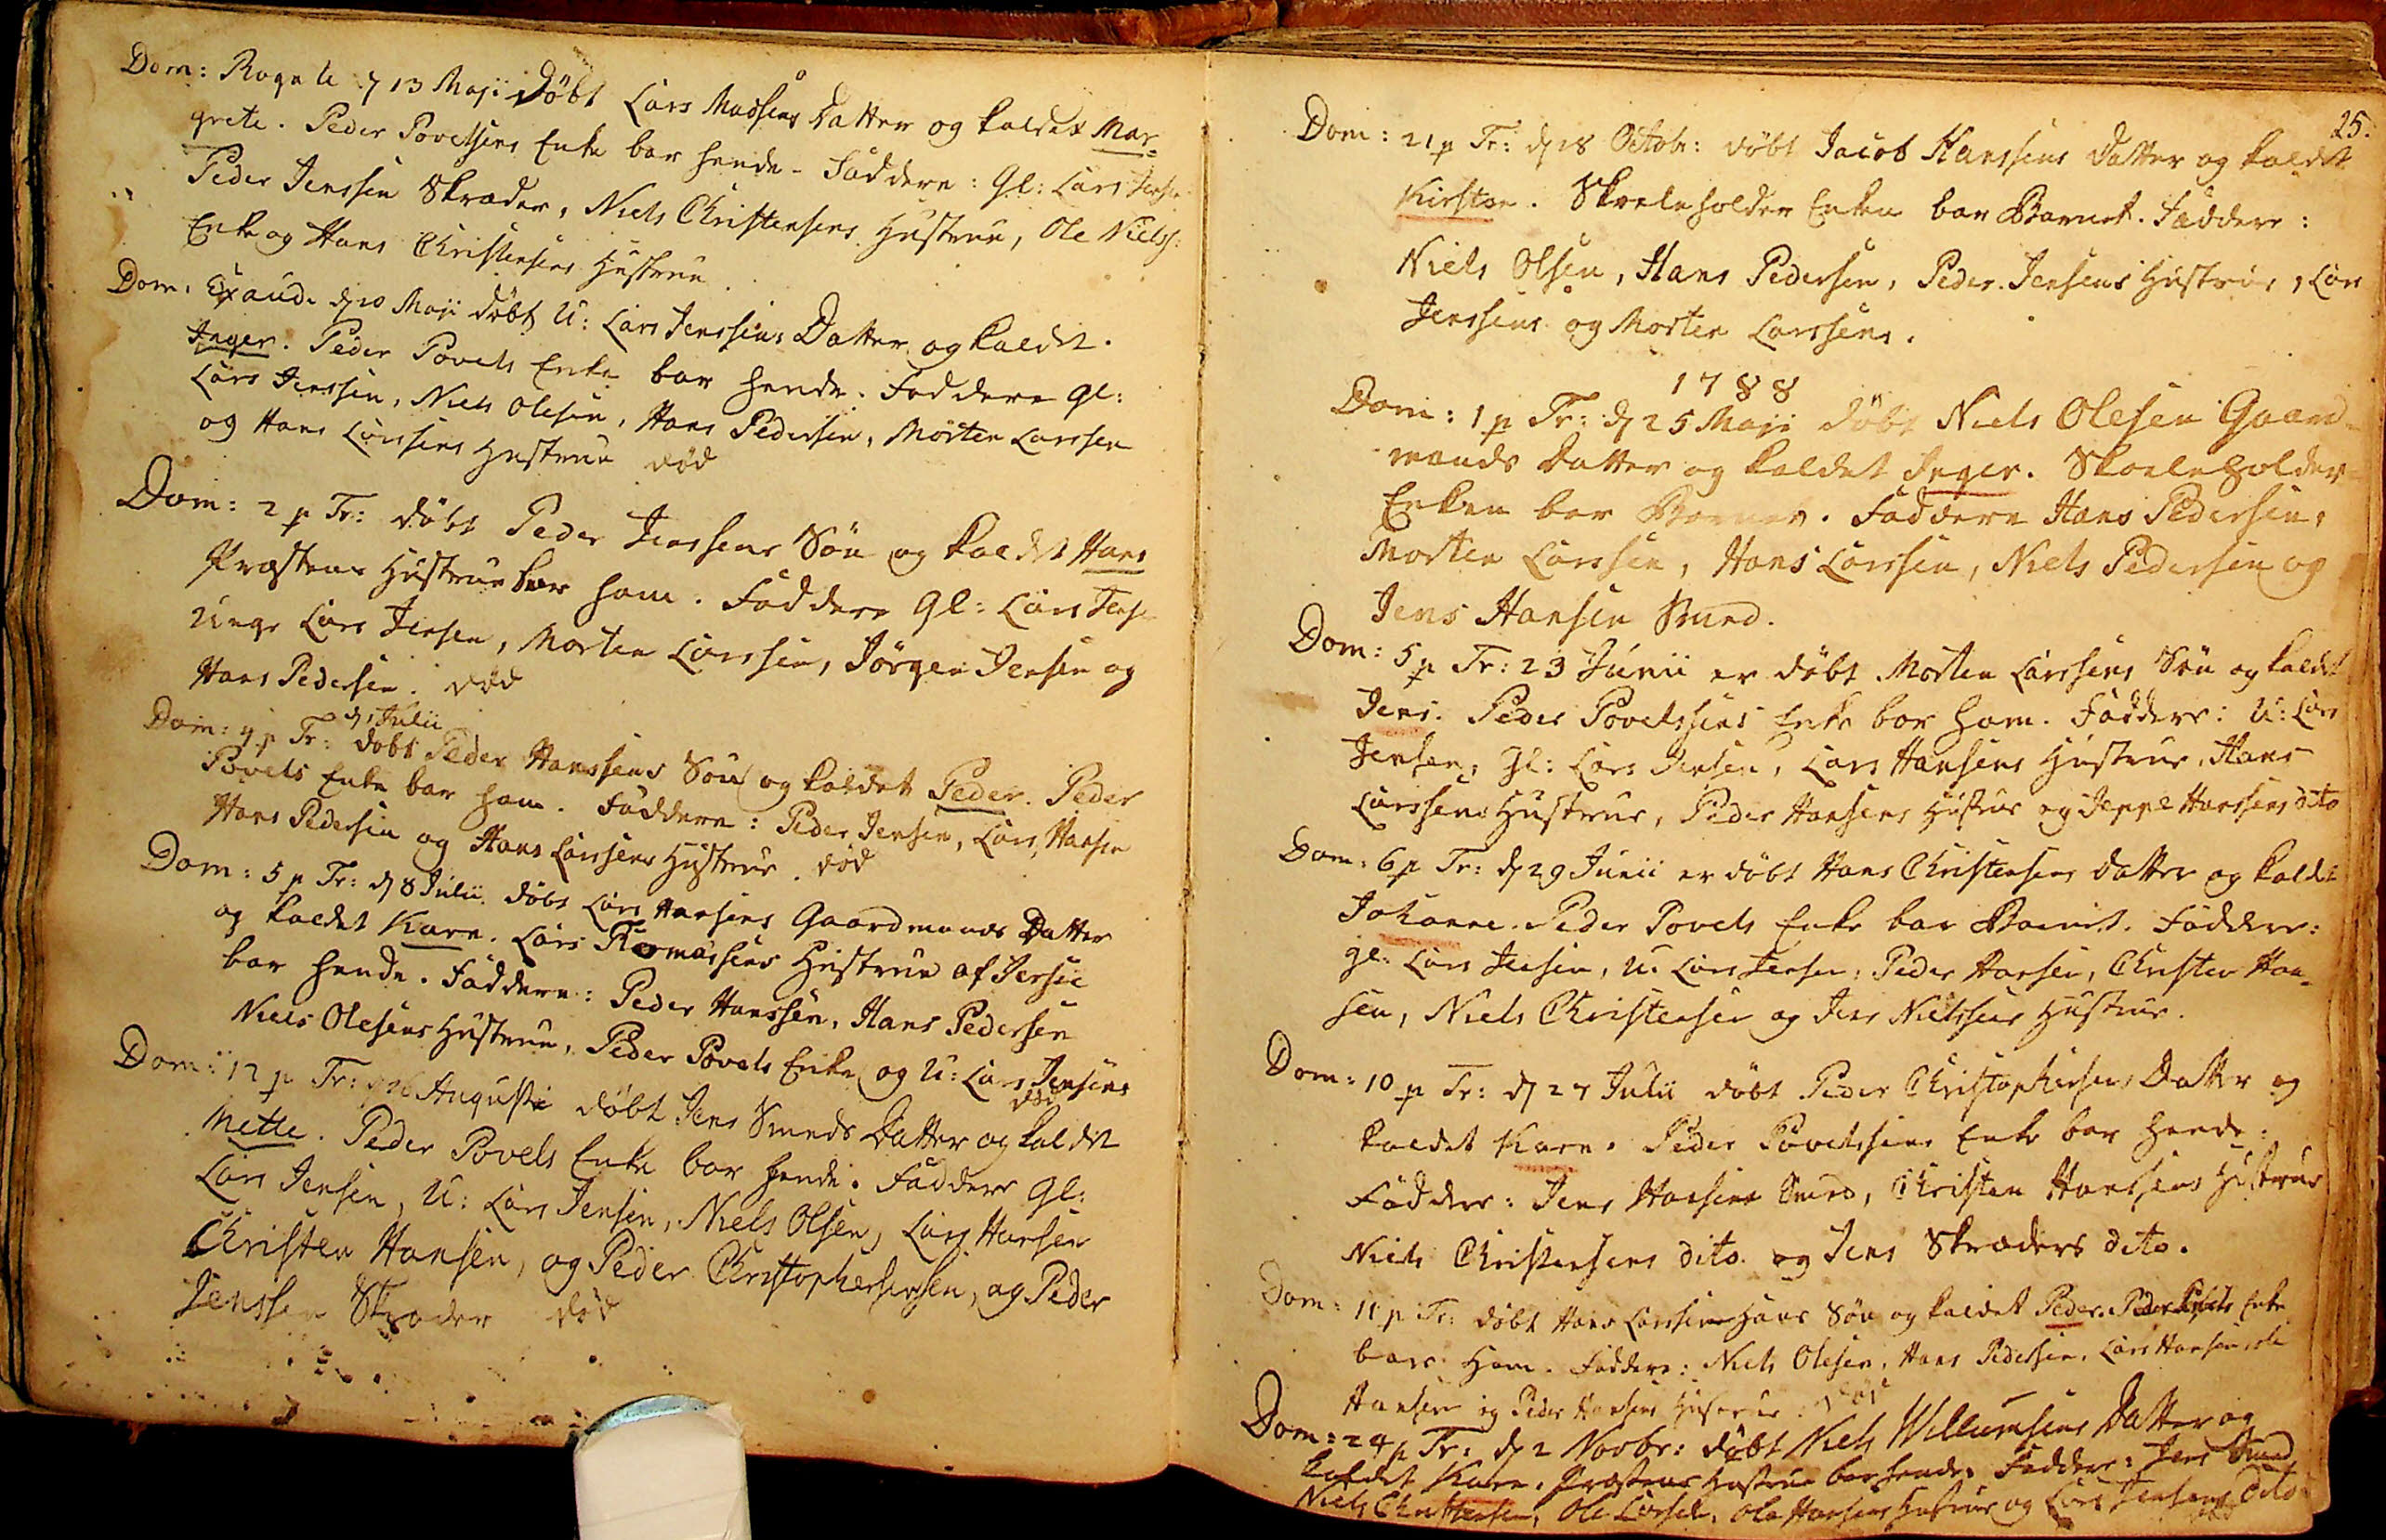Dom: Rogate d 13 Maji døbt Lars Madsens Datter og kaldet Mar¬
grete. Peder Povelsens Enke bar hende. Faddere: Gl:Lars Jensen
Peder Jenssen Skræder, Niels Christensens Hustrue, Ole Nielss:
Enke og Hans Christensens Hustrue.
Dom: Exaudi d 20 Maji døbt U: Lars Jenssens Datter og kaldet
Inger. Peder Povels Enke bar hende. Faddere Gl:
Lars Jenssen, Niels Olesen, Hans Pedersen, Morten Larssen
og Hans Larssens Hustrue død
Dom: 2 p Tr: døbt Peder Jenssens Søn og kaldet Hans
Præstens Hustrue bar ham. Faddere Gl: Lars Jensen
Unge Lars Jensen, Morten Larssen, Jørgen Jensen og
Hans Pedersen. død
d 1 Julii
Dom: 4 p Tr: døbt Peder Hanssens Søn og kaldet Peder. Peder
Povels Enke bar ham. Faddere: Peder Jensen, Lars Hansen
Hans Pedersen og Hans Larssens Hustrue. død
Dom: 5 p Tr: d 8 Julii døbt Lars Hansens Gaardmands Datter
og kaldet Karn. Lars Thomæsens Hustrue af Jersie
bar hende. Faddere: Peder Hanssen, Hans Pedersen
Niels Olesens Hustrue, Peder Povels Enke og U: Lars Jensens
død
Dom: 12 p Tr: d 26 Augusti døbt Jens Smeds Datter og kaldet
Mette. Peder Povels Enke bar hende. Faddere Gl:
Lars Jensen, U: Lars Jensen, Niels Olsen, Lars Hansen
Christen Hansen, og Peder Christophersen, og Peder
Jenssen Skræder død
25.
Dom: 21 p Tr: d 28 Octobr: døbt Jacob Hanssens Datter og kaldet
Kirsten. Skoleholder Enken bar Barnet. Faddere:
Niels Olsen, Hans Pedersen, Peder Jensens Hustrue, Lars
Jenssens og Morten Larssens.
1788
Dom: 1 p Tr: d 25 Maji døbt Niels Olesen Gaard¬
mands Datter og kaldet Inger. Skoleholder
Enken bar Barnet. Faddere Hans Pedersen,
Morten Larssen, Hans Larssen, Niels Pedersen og
Jens Hansen Smed.
Dom: 5 p Tr: 23 Junii er døbt Morten Larssens Søn og kaldet
Jens. Peder Povelsens Enke bar ham. Faddere: U: Lars
Jensen, Gl: Lars Jensen, Lars Hansens Hustrue, Hans
Larssens Hustrue, Peder Hansens Hustrue og Jeppe Hanssens dito
Dom: 6 p Tr: d 29 Junii er døbt Hans Christensens Datter og kaldet
Johanne. Peder Povels Enke bar Barnet. Faddere:
gl: Lars Jensen, U: Lars Jensen, Peder Hansen, Christen Han¬
sen, Niels Christensen og Jens Nielssens Hustrue.
Dom: 10 p Tr: d 27 Julii døbt Peder Christophersens Datter og
kaldet Karn. Peder Povelsens Enke bar hende.
Faddere: Jens Hansen Smed, Christen Hanssens Hustrue
Niels Christensens dito og Jens Skræders dito.
Dom: 11 p Tr: døbt Hans Larssen Hans Søn og kaldet Peder. Peder Povels Enke
bar Ham. Faddere: Niels Olesen, Hans Pedersen, Lars Hansen, Ole
Hansen og Peder Hansens Hustrue: død
Dom: 24 p Tr: d 2 Novbr: døbt Niels Willumsens Datter og
kaldet Karn. Præstens Hustrue bar hende: Faddere: Jens Smed
Niels Christensen, Ole Larsen, Ole Hansens Hustrue og Lars Jensens dito
død
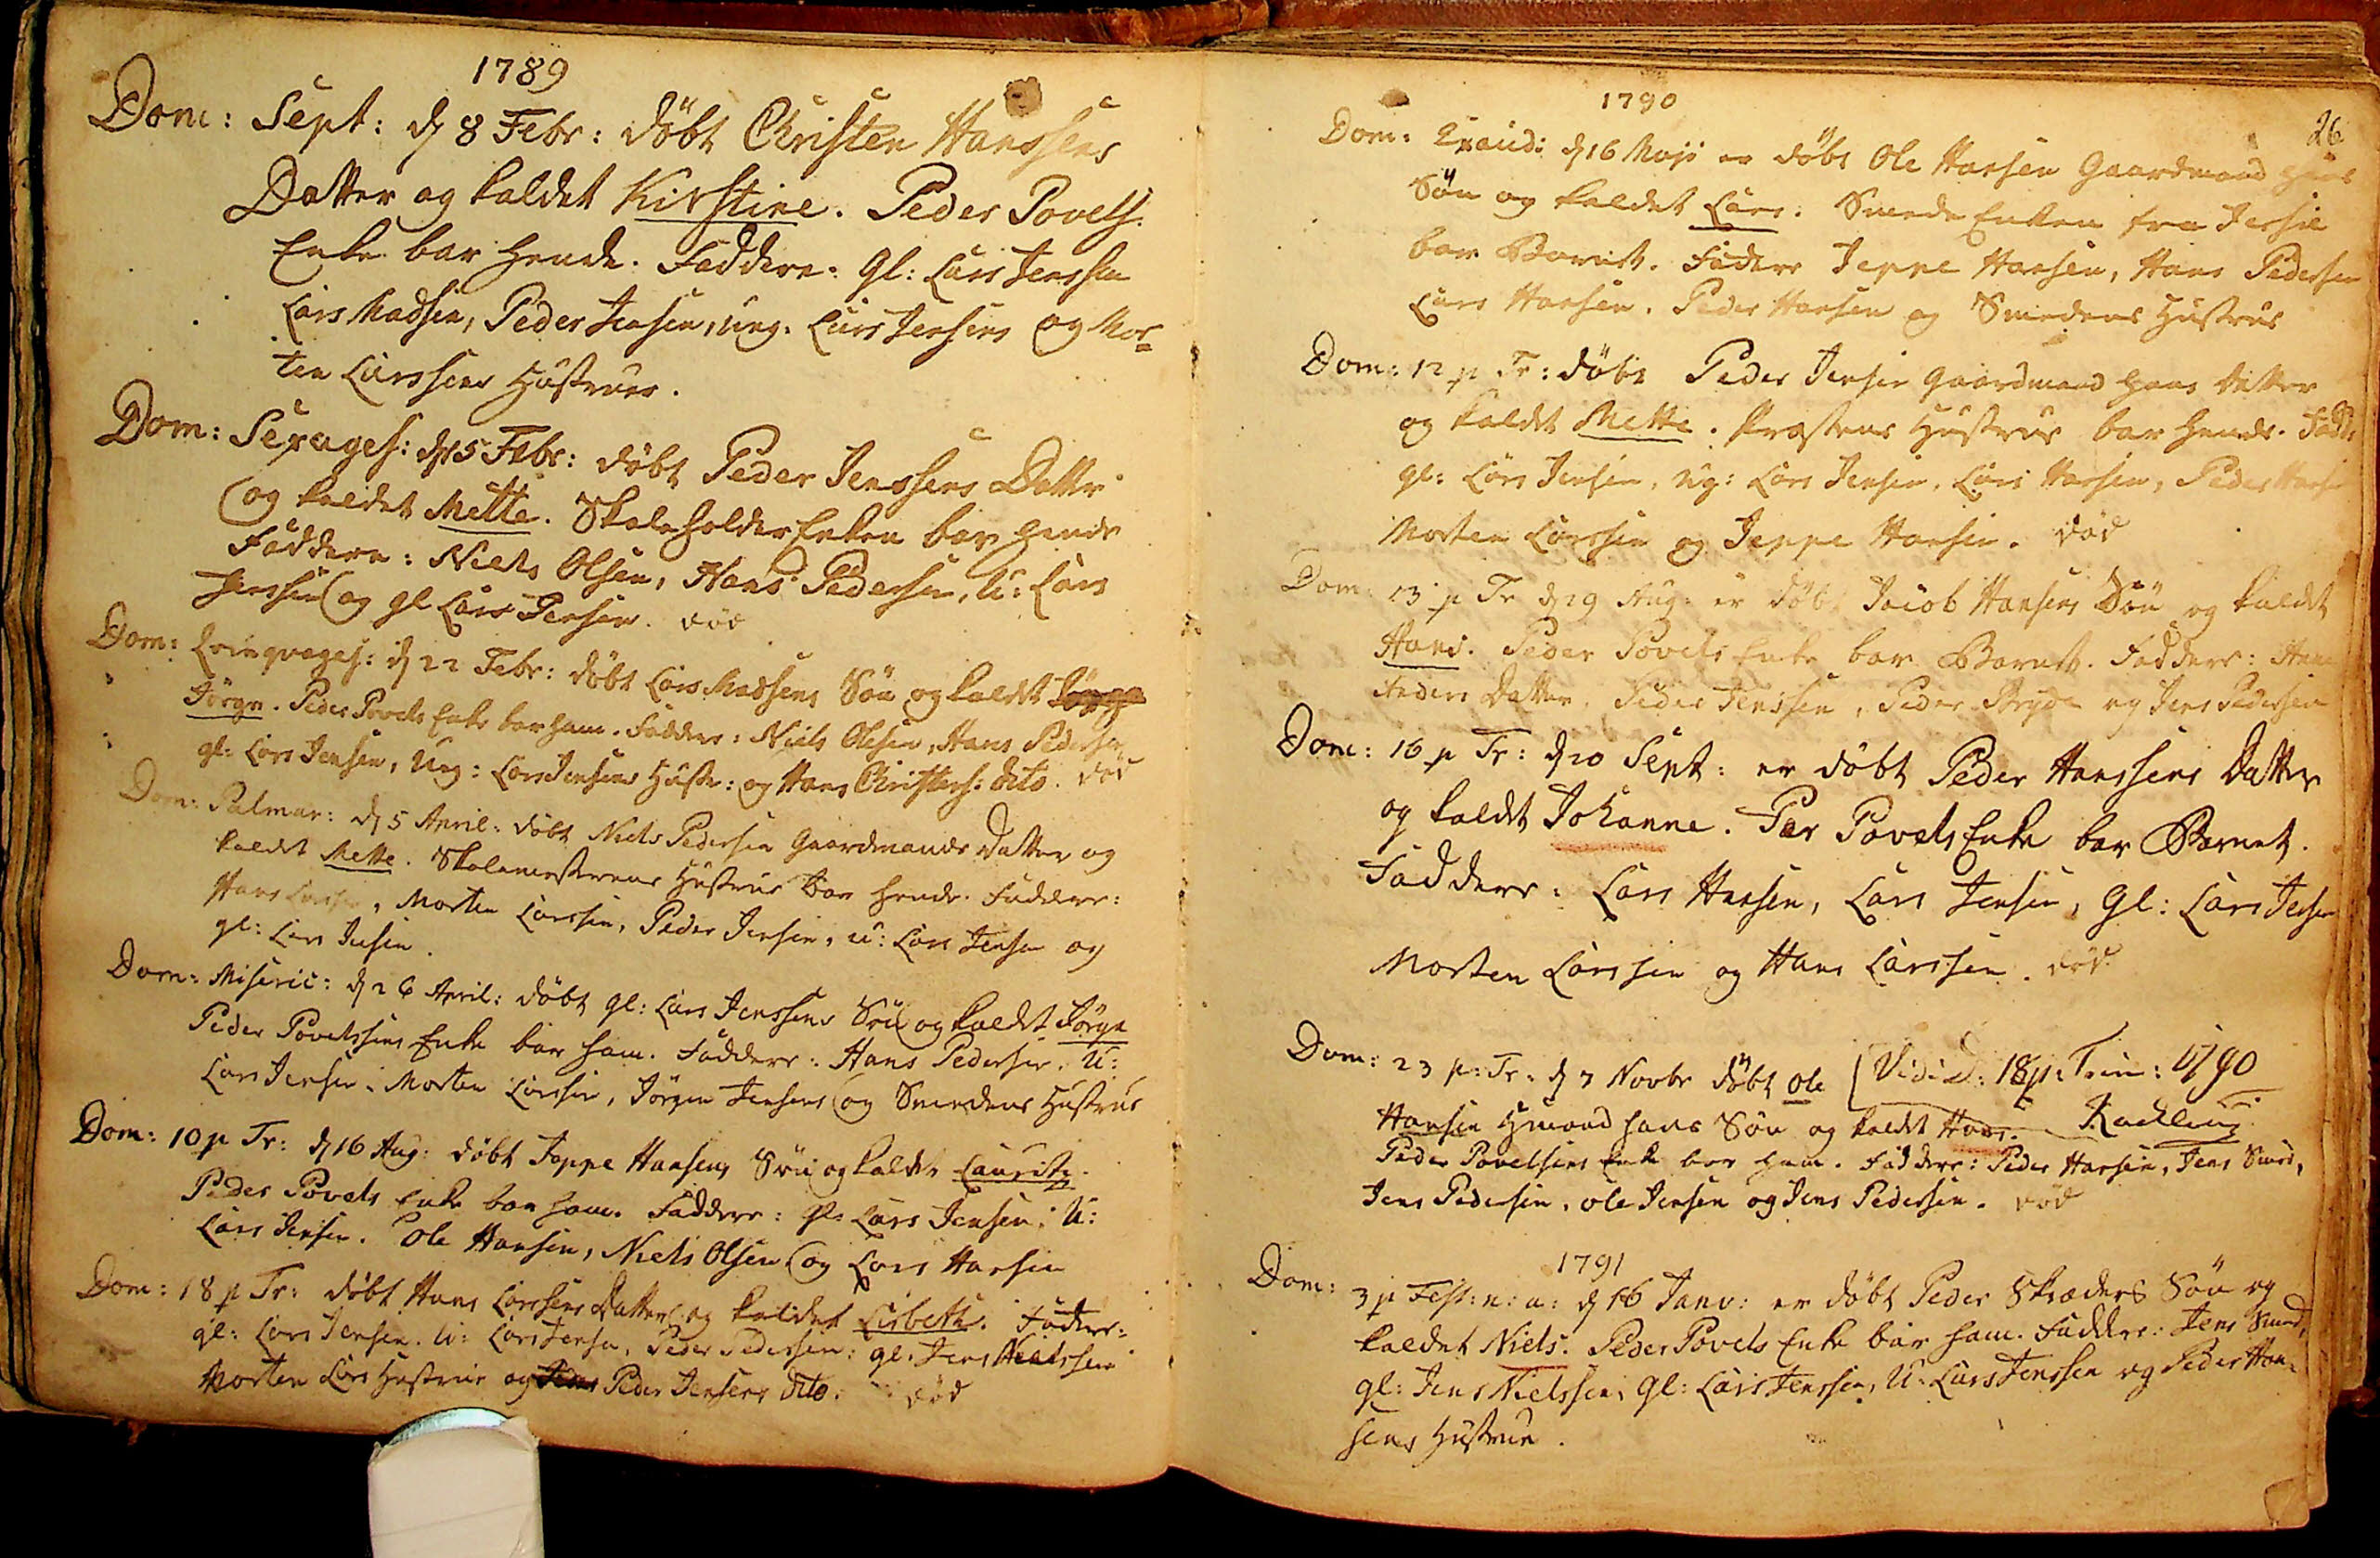1789
Dom: Sept: d 8 Febr: døbt Christen Hanssens
Datter og kaldet Kirstine. Peder Povels:
Enke bar hende. Faddere: Gl: Lars Jenssen
Lars Madsen, Peder Jensen, ung: Lars Jensens og Mor¬
ten Larssens Hustruer.
Dom: Sexages: d 15 Febr: døbt Peder Jenssens Datter
og kaldet Mette. Skoleholder Enken bar hende
Faddere: Niels Olsen, Hans Pedersen, U: Lars
Jenssen og gl Lars Jensen. død
Dom: Qvinqvages: d 22 Febr: døbt Lars Madsens Søn og kaldet Jørgn
Jørgn. Peder Povels Enke bar ham. Faddere: Niels Olesen, Hans Pedersen
gl: Lars Jensen, Ung: Lars Jensens Hustr: og Hans Christens: dito. død
Dom: Palmar: d 5 April: døbt Niels Pedersen Gaardmands Datter og
kaldet Mette. Skolemesterens Hustrue bar hende. Faddere:
Hans Larssen, Morten Larssen, Peder Jensen, u: Lars Jensen og
gl: Lars Jensen.
Dom: Miseric: d 26 April: døbt gl: Lars Jenssens Søn og kaldet Jørgn
Peder Povelsens Enke bar ham. Faddere: Hans Pedersen, U:
Lars Jensen, Morten Larssen, Jørgen Jensens og Smedens Hustrue
Dom: 10 p Tr: d 16 Aug: døbt Jeppe Hansens Søn og kaldet Lauritz.
Peder Povels Enke bar ham. Faddere: gl: Lars Jensen, U:
Lars Jensen. Ole Hansen, Niels Olsen og Lars Hansen
Dom: 18 p Tr: døbt Hans Larssens Datter og kaldet Lisbeth. Fadere:
gl: Lars Jensen. U: Lars Jensen, Peder Pedersen: gl: Jens Nielssen
Morten Lars Hustrue og Peder Peder Jensens dito. død
26.
1790
Dom: Exaudi: d 16 Maji er døbt Ole Hansen Gaardmand Hans
Søn og kaldet Lars. Smede Enken fra Jersie
bar Barnet. Fadere Jeppe Hansen, Hans Pedersen
Lars Hansen, Peder Hansen og Smedens Hustrue
Dom: 12 p Tr: døbt Peder Jensen Gaardmand hans Datter
og kaldet Mette. Præstens Hustrue bar hende. Fadd:
gl: Lars Jensen, ug: Lars Jensen, Lars Hansen, Peder Hansen
Morten Larssen og Jeppe Hansen. død
Dom: 13 p Tr: d 29 Aug: er døbt Jacob Hansens Søn og kaldet
Hans. Peder Povels Enke bar Barnet. Faddere: Anne
Anders Datter, Peder Jenssen, Peder Bryde og Jens Pedersen
Dom: 16 p Tr: d 20 Sept: er døbt Peder Hanssens Datter
og kaldet Johanne. Per Povels Enke bar Barnet.
Faddere: Lars Hansen, Lars Jensen, Gl: Lars Jensen
Morten Larssen og Hans Larssen. død
Dom: 23 p: tr: d 7 Novbr døbt Ole Vidi d: 18p:Trin:1790
Hansen Hmand hans Søn og kaldet Hans. Rachling
Peder Povelsens Enke bar ham.Faddere:Peder Hansen, Jens Smed,
Jens Pedersen, Ole Jensen og Jens Pedersen. død
1791
Dom: 3 p Fest: n: a: d 16 Janu: er døbt Peder Skræders Søn og
kaldet Niels. Peder Povels Enke bar ham. Faddere: Jens Smed
gl:Jens Nielsen, gl:Lars Jenssen, U:Lars Jenssen og Peder Han¬
sens Hustrue.